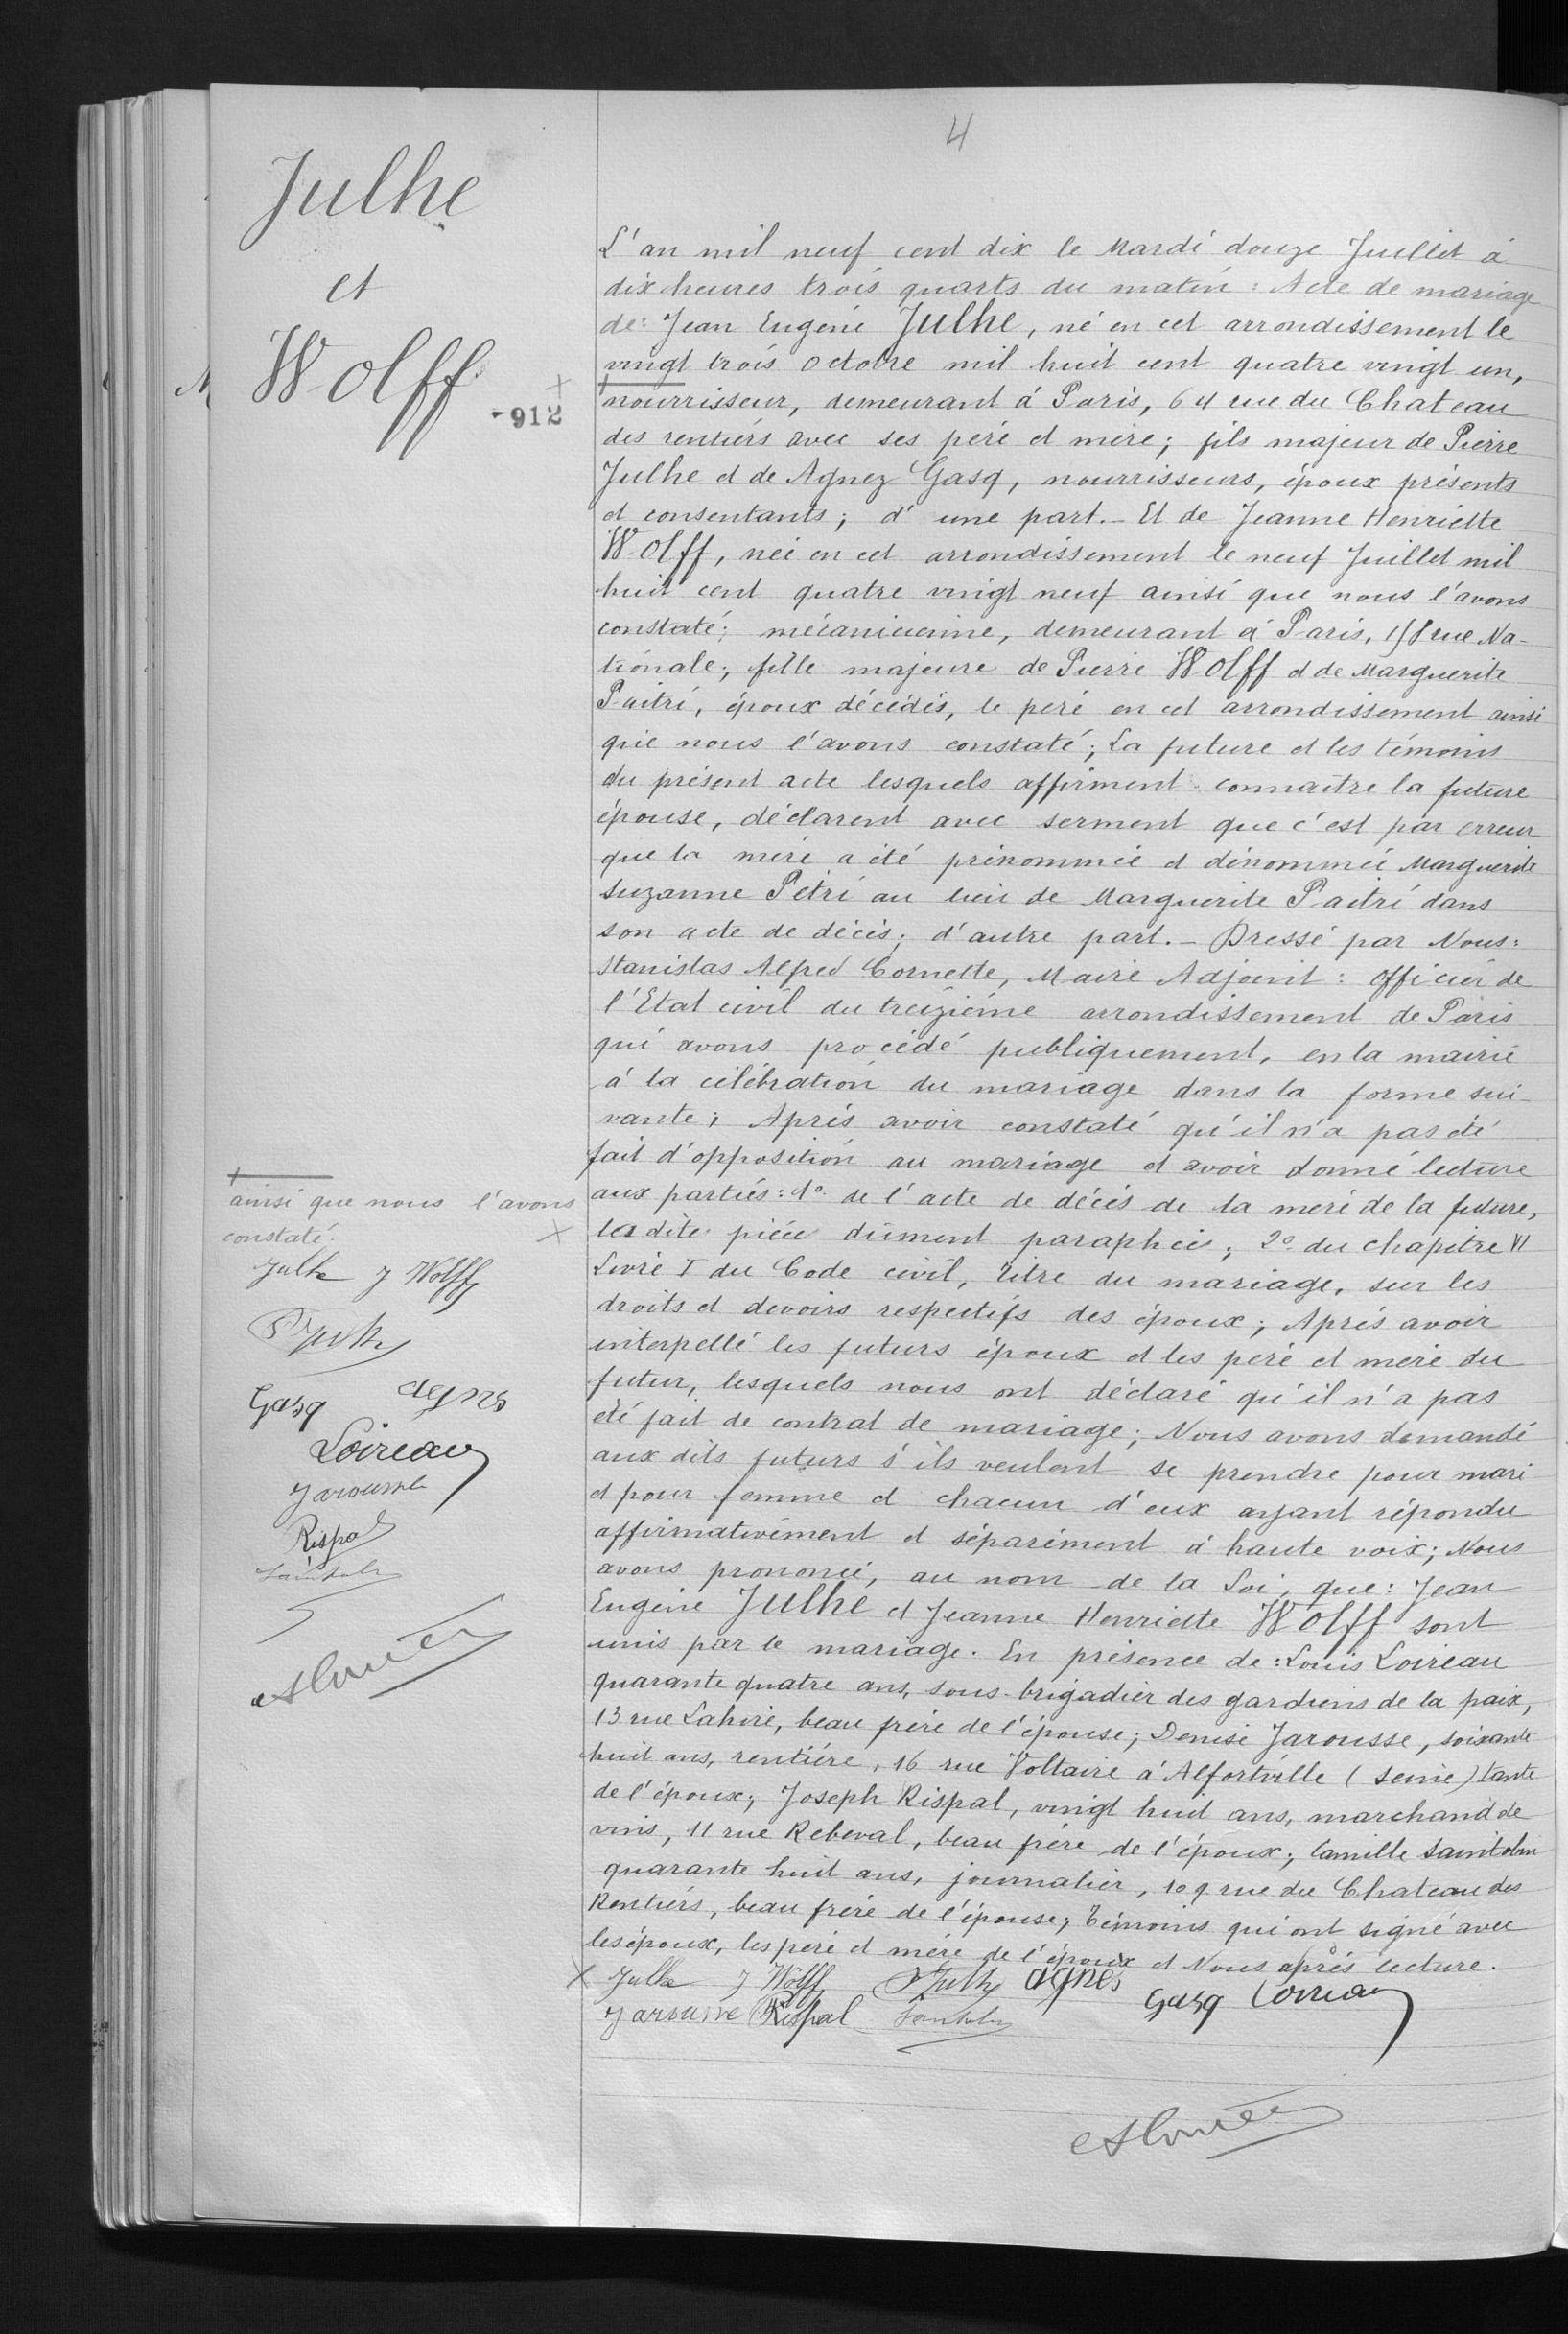Julhe
et
Wolff
L'an mil neuf cent dix le mardi douze Juillet à
dix heures trois quarts du matin: Acte de mariage
de Jean Eugénie Julhe, né en cet arrondissement le
vingt trois octobre mil huit cent quatre vingt un,
nourrisseur, demeurant à Paris, 64 rue du Chateau
des rentiers aves ses père et mère; fils majeur de Pierre
Julhe et de Agnez Gasq, nourrisseurs, époux présents
et consentants; d'une part.-Et de Jeanne Henriette
Wolff, née en cet arrondissement le neuf Juillet mil
huit cent quatre vingt neuf ainsi que nous l'avons
constaté: mécanicienne, demeurant à Paris, 158 rue Na-
tionale; fille majeure de Pierre Holff et de Marguerite
Paitri, époux décédés, le père en cet arrondissement ainsi
que nous l'avons constaté; La future et les témoins
du présent acte lesquels affirment connaître la future
épouse, déclarent avec serment que c'est par erreur
que la mère a été prénommée et dénommée Marguerite
Suzanne Pétri au lieu de Marguerite Paitri dans
son acte de décès; d'autre part.-Dressé par Nous:
Stanislas Alfred Cornette, Maire Adjoint: officier de
l'Etat civil du treizième arrondissement de Paris
qui avons procédé publiquement, en la mairie
à la célébration du mariage dans la forme sui-
vante; Après avoir constaté qu'il n'a pas été
fait d'opposition au mariage et avoir donné lecture
aux parties: 1° de l'acte de décès de la mère de la future,
ladite pièce dûment paraphées; 2° du chapitre VI
Livre I du Code civil, titre du mariage, sur les
droits et devoirs respectifs des époux; Après avoir
interpellé les futurs époux et les père et mère du
futur, lesquels nous ont déclaré qu'il n'a pas
été fait de contrat de mariage; Nous avons demandé
aux dits futurs s'ils veulent se prendre pour mari
et pour femme et chacun d'eux ayant répondu
affirmativement et séparément à haute voix; Nous
avons prononcé au nom de la Loi, que: Jean
Eugénie Julhé et Jeanne Henriette Wolff sont
unis par le mariage. En présence de: Louis Lorreau
quarante quatre ans, sous-brigadier des gardiens de la paix,
13 rue Lahire, beau père de l'épouse; Denise Jarousse, soixante
huit ans, rentière, 16 rue Voltaire à Alfortville (Seine) tante
de l'époux; Joseph Rispal, vingt huit ans, marchand de
vins, 11 rue Rebeval, beau père de l'époux; Camille Saint Denis
quarante huit ans, journalier, 109 rue du Chateau des
Rentiers, beau frère de l'épouse; témoins qui ont signé avec
les époux, les père et mère de l'épouse et Nous après lecture
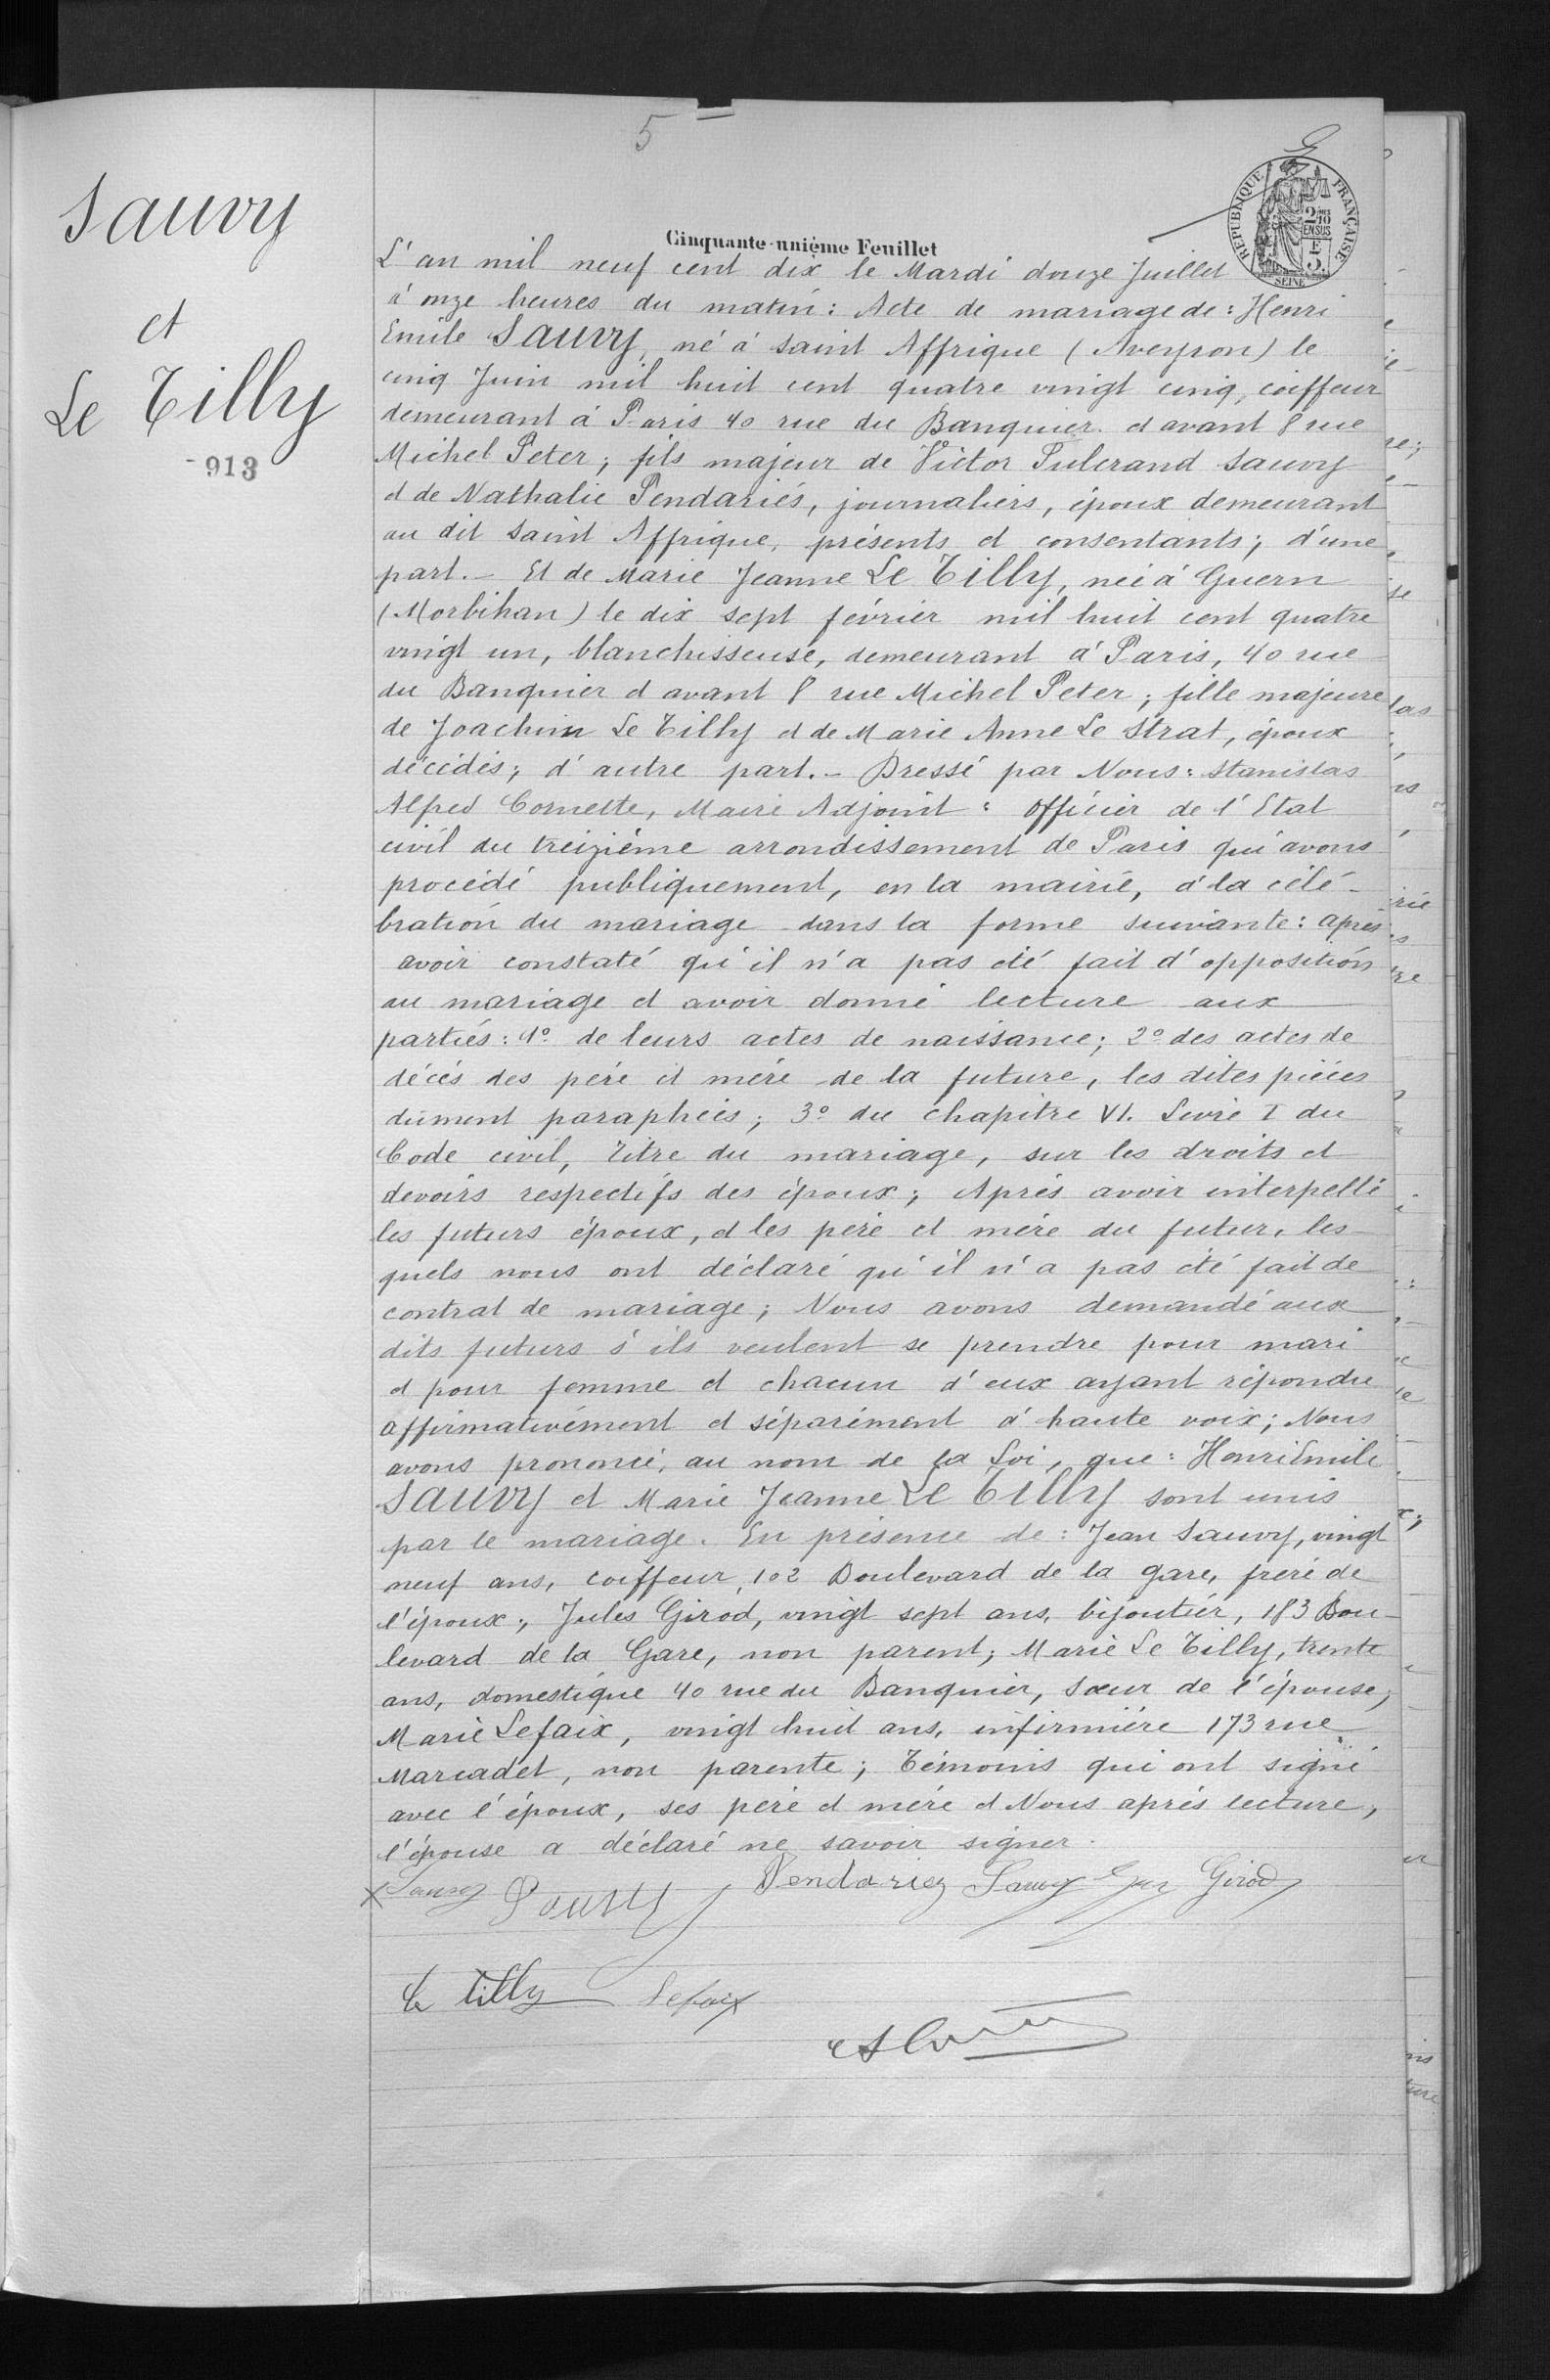Sauvy
et
Le Tilly
913
L'an mil neuf cent dix le Mardi douze juillet
à onze heures du matin: Acte de mariage de: Henri
Emile Sauvy, né à Saint Affrique (Aveyron) le
cinq Juin mil huit cent quatre vingt cinq, coiffeur
demeurant à Paris 40 rue des Banquier et avant 8 rue
Michel Peter; fils majeur de Victor Pulcrand Sauvy
et de Nathalie Pendariès, journaliers, époux demeurant
au dit Saint Affrique, présents et consentants; d'une
part.-Et de Marie Jeanne Le Tilly, née à Guern
(Morbihan) le dix sept février mil huit cent quatre
vingt un, blanchisseuse, demeurant à PAris, 40 rue
du Banquier et avant 8 rue Michel Peter; fille majeure
de Joachim le Tilly et de Marie Anne Le Strat, époux
décédés; d'autre part.-Dressé par Nous: Stanislas
Alfred Cornette, Maire Adjoint: Officier de l'Etat
civil du treizième arrondissement de Paris qui avons
procédé publiquement en la mairie à la célé-
bration du mariage dans la forme suivante: après
avoir constaté qu'il n'a pas été fait d'oppositio
au mariage et avoir donné lecture aux
parties: 1° de leurs actes de naissance; 2° des actes de
décès des père et mère de la future, les dites pièces
dûment paraphées; 3° du chapitre VI. Livre I du
Code civil, Titre du mariage, sur les droits et
devoirs respectifs des époux; Après avoir interpellé
les futurs époux, et les père et mère du futur. les
quels nous ont déclaré qu'il n'a pas été fait de
contrat de mariage; Nous avons demandé aux
dits futurs s'ils veulent se prendre pour mai
et pour femme et chacun d'eux ayant répondu
affirmativement et séparément à haute voix; Nous
avons prononcé au nom de la Loi que: Henri Emile
Sauvy et Marie Jeanne Le Tilly sont unis
par le mariage. En présence de: Jean Sauvy, vingt
neuf ans, coiffeur, 102 Boulevard de la Gare, frère de
l'époux; Jules Girod, vingt sept ans, bijoutier, 183 Bou-
levard de la Gare, non parent; Marie Le Tilly, trente
ans, domestiue 40 rue du Banquier, soeur de l'épouse,
Marie Lefaix, vingt huit ans, infirmière 173 rue
Marcadet, non parente; témoins qui ont signé
avec l'époux, ses père et mère et Nous après lecture,
l'épouse a déclaré ne savoir signer.